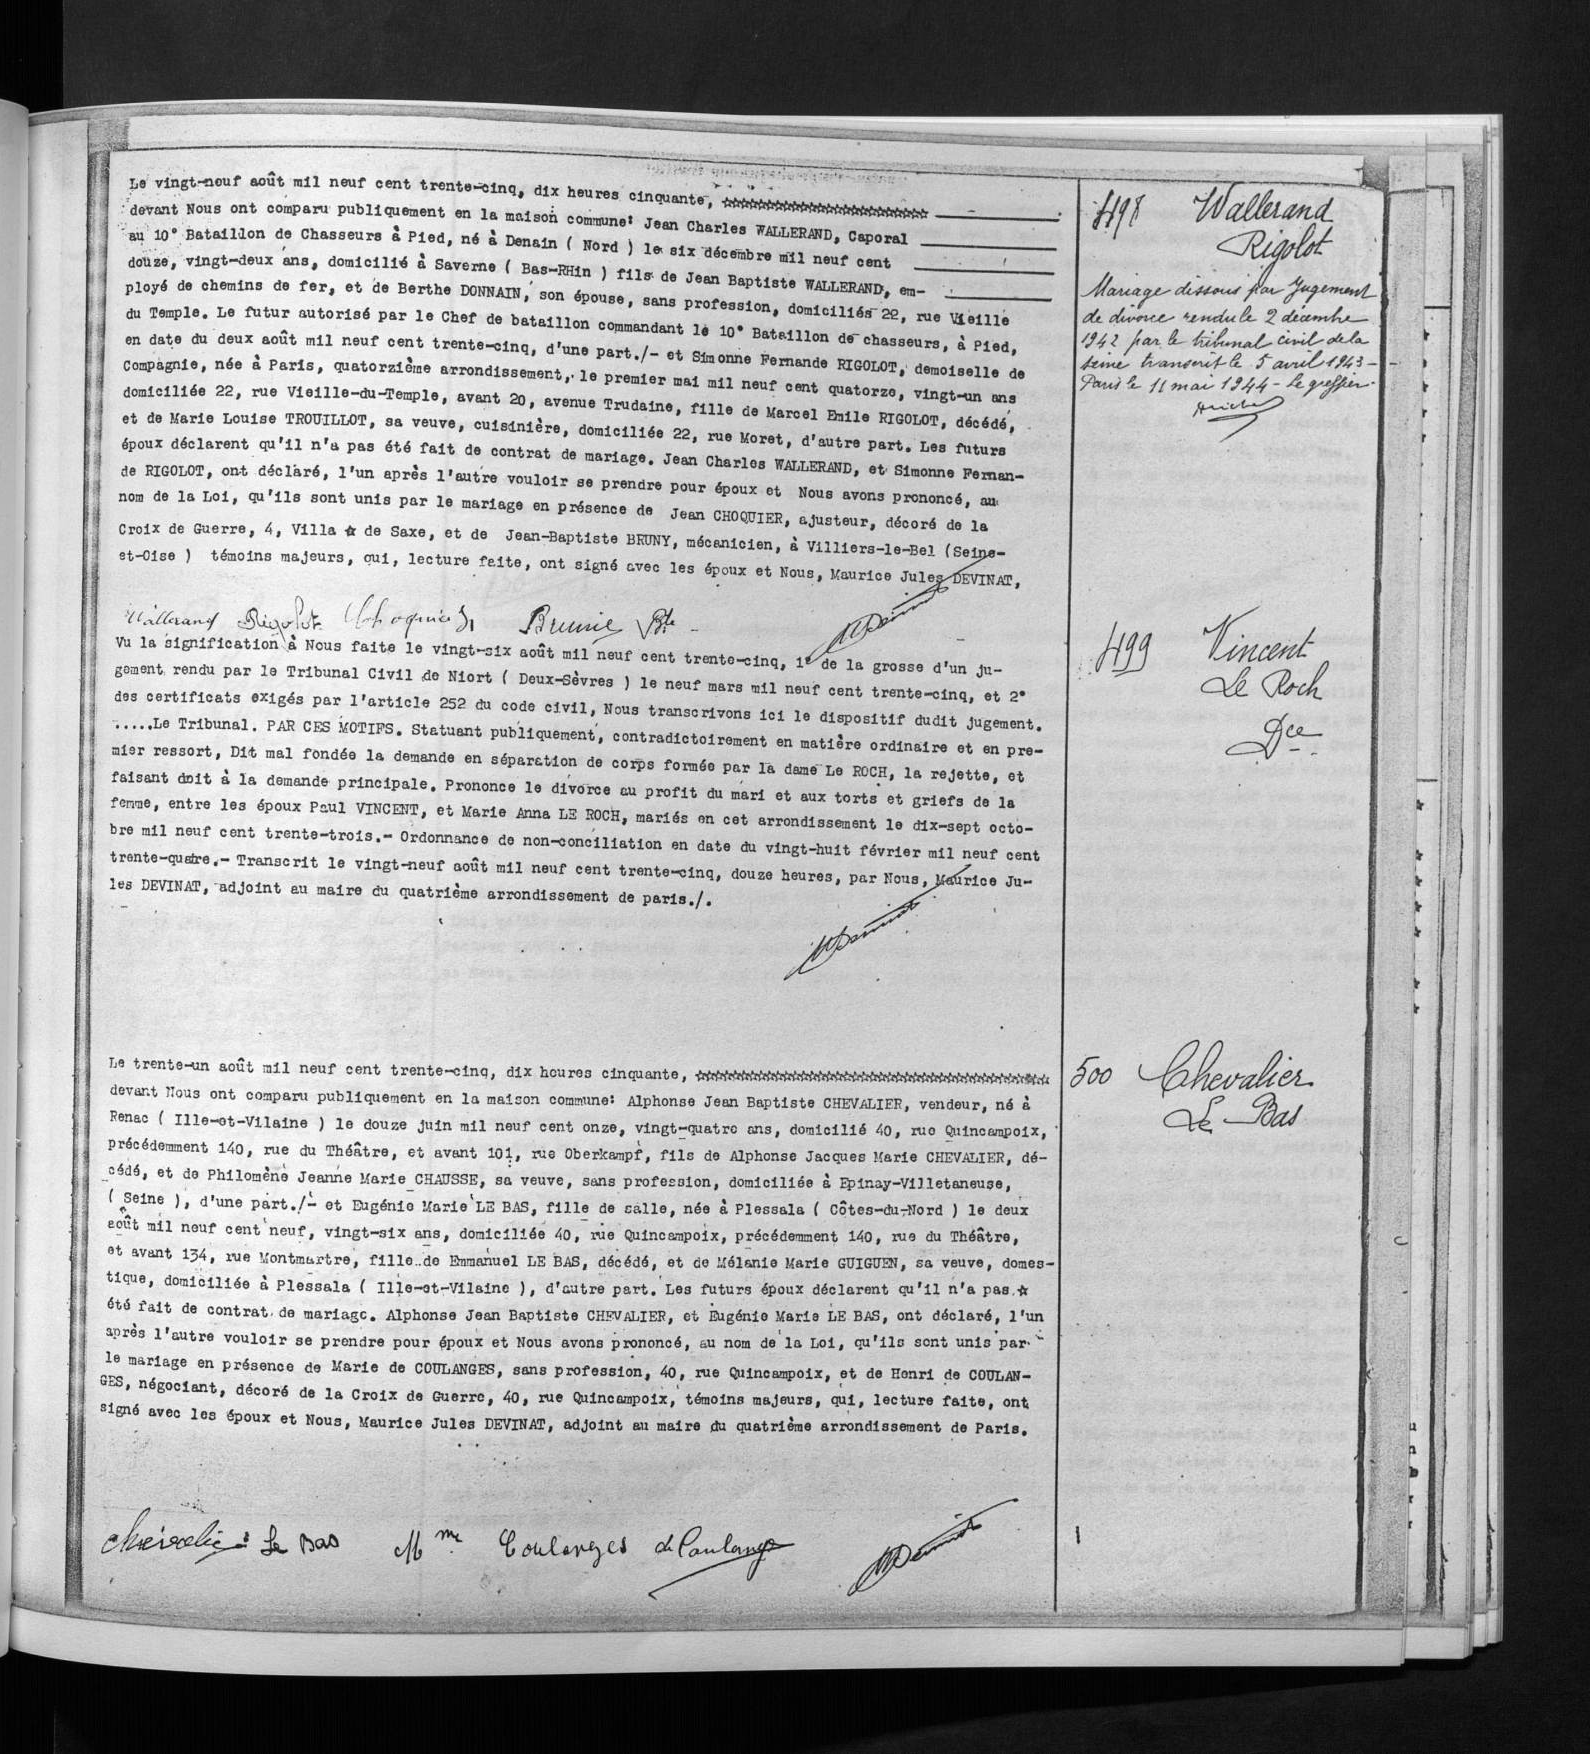Le vingt-neuf août mil neuf cent trente-cinq, dix heures cinquante, ****-
devant Nous ont comparu publiquement en la maison commune: Jean Charles WALLERAND, Caporal -
au 10° Bataillon de Chasseurs à Pied, né à Denain (Nord) le six décembre mil neuf cent -
douze, vingt-deux ans, domicilié à Saverne (Bas-RHin) fils de Jean Baptiste WALLERAND, em -
ployé de chemins de fer, et de Berthe DONNAIN, son épouse, sans profession, domiciliés 22, rue Vieille
du Temple. Le futur autorisé par le Chef de bataillon commandant le 10° Bataillon de chassuers, à Pied,
en date du deux août mil neuf cent trente-cinq, d'une part ./-et Simone Fernande RIGOLOT, demoiselle de
Compagnie, née à Paris, quatorzième arrondissement, le premier mai mil neuf cent quatorze, vingt-un ans
domicilié 22, rue Vieille-du-Temple, avant 20, avenue Trudaine, fille de Marcel Emile RIGOLOT, décédé,
et de Marie Louise TROUILLOT, sa veuve, cuisinière, domiciliée 22, rue Moret, d'autre part. Les futurs
époux déclarent qu'il n'a pas été fait de contrat de mariage. Jean Charles WALLERAND, et Simon Fernan-
de RIGOLOT, ont déclaré, l'un après l'autre vouloir se prendre pour époux et Nous avons prononcé, au
nom de la Loi, qu'ils sont unis par le mariage en présence de Jean CHOQUIER, ajusteur, décoré de la
Croix de Guerre, 4, Villa * de Saxe, et de Jean-Baptiste BRUNY, mécanicien, à Villiers-le-Bel (Seine-
et-Oise à témoins majeurs, qui, lecture faite, ont signé avec les époux et Nous, Maurice Jules DEVINAT,
Vu la signification à Nous faite le vingt-six août mil neuf cent trente-cinq, 1° de la grosse d'un ju-
gement rendu par le Tribunal Civil de Niort (Deux Sèvres) le neuf mars mil neuf cent trente-cinq, et 2°
des certificats exigés par l'article 252 du code civil, Nous transcrivons ici le dispositif dudit jugement.
..... Le Tribunal. PAR CES MOTIFS. Statuant publiquement, contradictoirement en matière ordinaire et en pre-
mier ressort, Dit mal fondée la demande en séparation de corps formée par la dame LE ROCH, la rejette, et
faisant droit à la demande principale. Pronnonce le divorce au profit du mari et aux torts et griefs de la
femme, entre les époux Paul VINCENT, et Marie Anna LE ROCH, mariés en cet arrondissement le dix-sept octo-
bre mil neuf cent trente-trois .-Ordonnance de non-conciciliation en date du vingt-huit février mil neuf cent
trente-quatre .-Transcrit le vingt-neuf août mil neuf cent trente-cinq, douze heures, par Nous, Maurice Ju-
les DEVINAT, adjoint au maire du quatrième arrondissement de paris ./.
Le trente-un août mil neuf cent trente-cinq, dix heures cinquante, ****
devant Nous ont comparu publiquement en la maison commune: Alphonse Jean Baptiste CHEVALIER, vendeur, né à
Renac (Ille-et-Vilaine) le douze juin mil neuf cent onze, vingt-quatre ans, domicilié 40, rue Quincampoix,
précédemment 140, rue tu Théâtre, et avant 101, rue Oberkampf, fils de Alphonse Jacques Marie CHEVALIER, dé-
cédé, et de Philomène Jeanne Marie CHAUSSE, sa veuve, sans profession, domiciliée à Epinay-Villetaneuse,
(Seine), d'une part ./-et Eugénie Marie LE BAS, fille de salle, née à Plessala (Côtes-du-Nord) le deux
août mil neuf cent neuf, vingt-six ans, domiciliée 40, rue Quincampoix, précédemment 140, rue du Théâtre,
et avant 134, rue Montmartre, fille de Emmanuel LE BAS, décédé, et de Mélanie Marie GUIGUEN, sa veuve, domes-
tique, domiciliée à Plessala (Ille-et-Vilaine), d'autre part. Les futurs époux déclarent qu'il n'a pas *
été fait de contrat de mariage. Alphonse Jean Baptiste CHEVALIER, et Eugénie Marie LE BAS, ont déclaré, l'un
après l'autre vouloir se prendre pour époux et Nous avons prononcé, au nom de la Loi, qu'ils sont unis par
le mariage en présence de Marie de COULANGES, sans profession, 40, rue Quincampoix, et de Henri de COULAN-
GES, négociant, décoré de la Croix de Guerre, 40, rue Quincampoix, témoins majeurs, qui, lecture faite, ont
signé avec les époux et Nous, Maurice Jules DEVINAT, adjoint au maire du quatrième arrondissement de Paris.